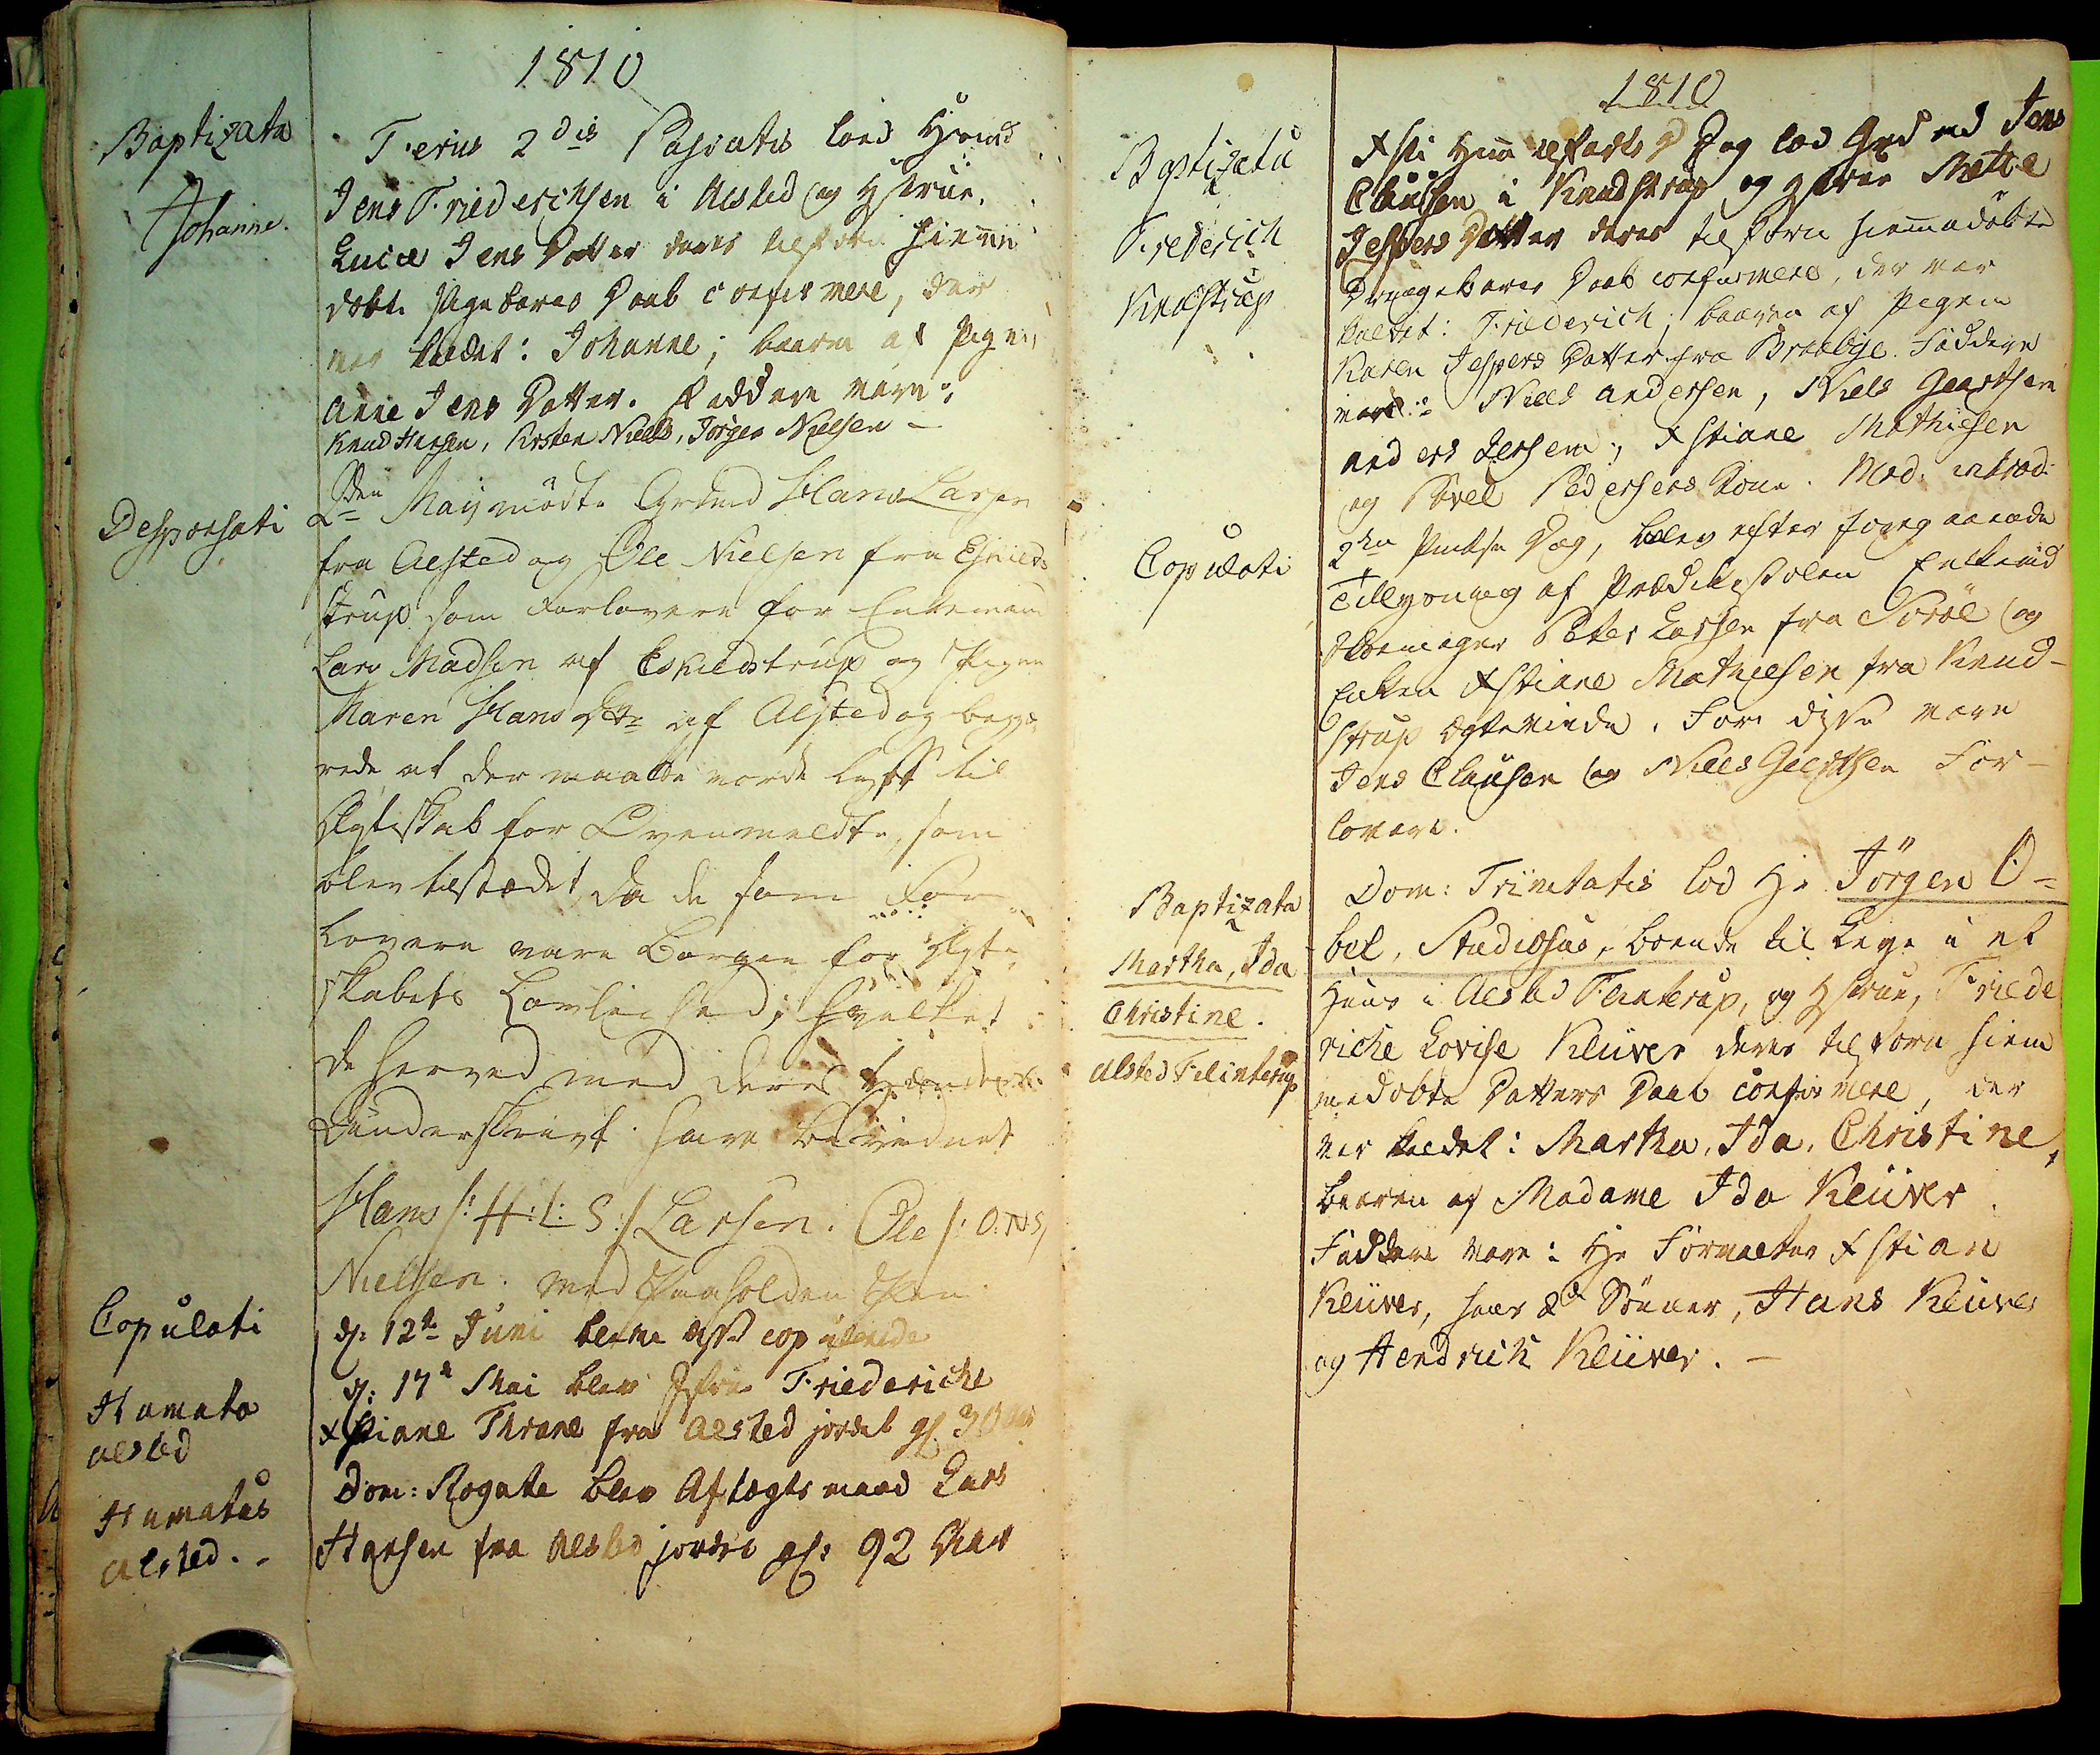Baptizata
Johanne
Desponsati
Copulati
Humata
Alsted
Hamatus
Alsted. -
1810
Feriis 2dis Pascatis loed Hsmd
Jens Friderichsen i Alsted og Hstrue.
Luice Jens Datter deres tilforn hiemme
døbet Pigebarns Daab confirmere, der
var kaldet: Johanne; baaren af Pigen
Anne Jens Datter. Faddere vare:
Knud Hansen, Krsten Niels, Jørgen Nielsen
2den Maij mødte Grdmd Hans Larsen
fra Alsted og Ole Nielsen fra Eskild¬
strup, som Forlovere for Enkemand
Lars Madsen af Eskildstrup og Pigen
Karen Hans Dtr af Alsted og begge¬
rede at der maatte vorde lyst til
Egteskab for Ovenmeldte, som
blev tilstædet da de som For¬
lovere vare Borgen for Ægte¬
skabets Lovlighed, hvilket
de herved med deres Hænders
Underskrivt have Bevidnet
Hans /: H: L: S :/ Larsen. Ole/: O: N. S/
Nielsen. med paaholden Pen
d: 12te Junii bleve disse copulerede
d: 17de Mai blev Jfrue Frideriches
Xstiane Thrane fra Alsted jordet gl 30 Aar
Dom: Rogate blev Aftægtsmand Lars
Hansen fra Alsted jordet gl: 92 Aar
Baptizatu
Frederich
Knudstrup
Copulati
Baptizata
Martha Ida
Christine
Alsted Flinterup
1810
Xsti Himmelfarts Dag lod Grdmd Jens
Claussen i Knudstyrup og Hstrue Mette
Jespers Datter deres tilforn hiemmedøbte
Drengebarns Daab confirmere, der var
kaldet: Friderich; baaren af Pigen
Karen Jespers Datter fra Braabye. Faddere
vare: Niels Anderssen, Niels Geertsen
Anders Jensen, Xstiane Mathisen
og Povel Pedersens Kone. Mod: introd:
2den Pindse Dag, blev efter foregaaende
Tillysning af Prædikestolen Enkemd
Skoemager Peter Larsen fra Sorøe og
Enken Xstiane Mathiesen fra Knud¬
strup Ægteviede. For disse vare
Jens Clausen og Niels Geertsen For¬
lovere.
Dom: Trinitatis lod Hr Jørgen O¬
bel Studiosus, boende til Leye i et
Huus i Alsted Flinterup, og Hstrue, Friede
riche Lovise Kluver deres tilforn hiem¬
medøbte Datters Daab confirmere, der
var kaldet: Martha, Ida, Christine,
baaren af Madame Ida Kluver
Faddere vare: Hr Forvalter Xstian
Kluver, hans 2de Sønner, Hans Kluver
og Hendrich Kluver.
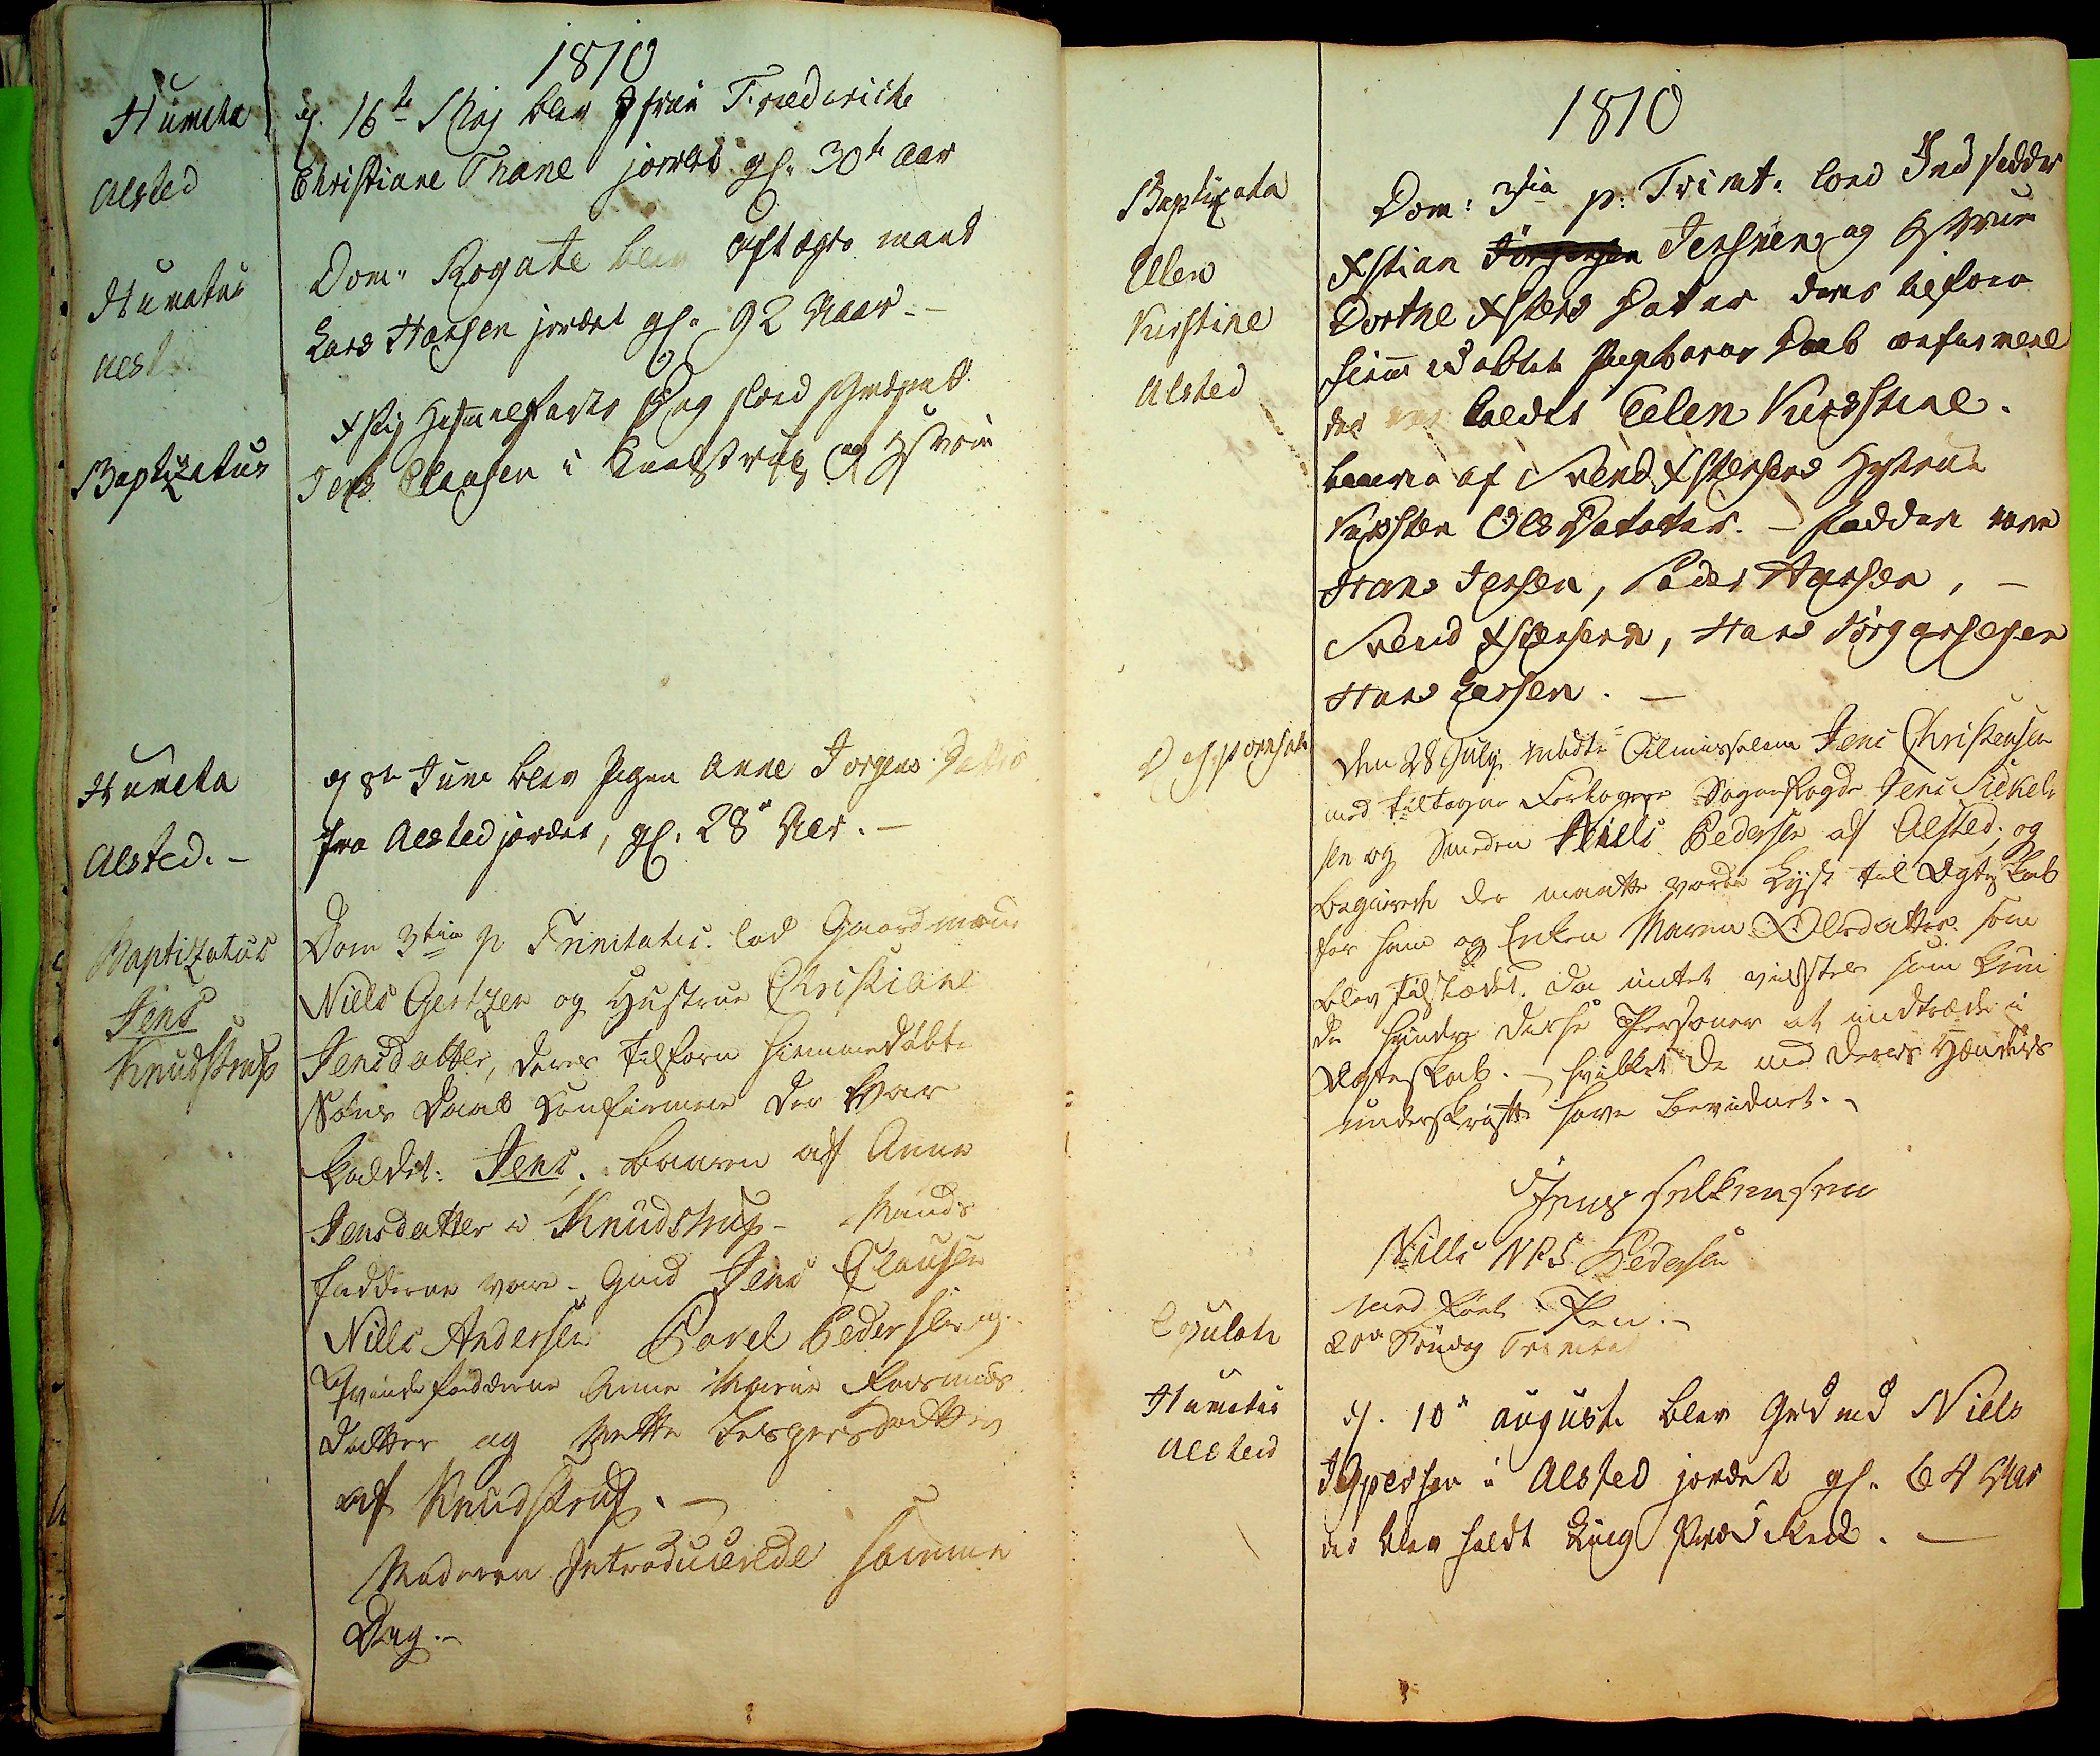Humata
Alsted
Humata
Alsted
Baptizatus
Humata
Alsted.
Baptizatus.
Jens
Knudstrup
1810
d. 16ta Maj blev Jfrue Frideriche
Christiane Thane jordet gl. 30t Aar.
Dom: Rogate blev Aftægtsmand
Lars Hansen jordet gl. 92 aar.
Xsti Himmelfarts Dag loed Grdmd
Jens Clausen i Knudstrup og Hstrue
d 8te Juni blev Pigen Anne Jørgens Datter
fra Alsted jordet gl. 28## Aar.
Dom 3tia p Trinitatis lod Gaardmand
Niels Gertzen og Hustrue Christiane
Jensdatter, deres tilforn hiemmedøbte
Søns Daab Confirmere der var
kaldet: Jens. baaren af Anne
Jensdatter i Knudstrup - Mands
Fadderne vare - Gmd Jens Clausen
Niels Andersen Povel Pedersen og
Qvinde Fadderne Anne Maren Rasmus
datter og Mette Jespersdatter
af Knudstrup.
Moderen Introducerede samme
Dag.
Baptizata
Ellen
Kirstine
Alsted
Desponsati
Copulati
Humatus
Alsted
1810
Dom: 3tia p: Trinit: loed Indsidder
Xstian Jørgensen Jensen og Hstrue
Dorthe Xstens Datter deres tilforn
shiemmedøbte Pigebarns Daab confirmere
det var kaldet Ellen Kirstine.
baaren af Svend Xstensens Hstrue
Kirsten Ols Datter. Faddere vare
Hans Jensen, Peder Hansen
Svend Xstensen, Hans Jørgensen
Hans Larsen.
Den 28 Julij mødte Almisselem Jens Christensen
med tiltagne Forlovere Sognefogde Jens Silken
sen og Smeden Niels Pedersen af Alsted og
begierede der maatte vorde Lyst til Ægteskab
for ham og Enken Maren Olsdatter, som
blev tilstædet, da intet vidste som kun
de hindre disse Personer at indtræde i
Ægteskab. hvilket de med deres Hænders
Underskrift have bevidnet.
Jens Silkensen
Niels NPS Pedersen
med ført Pen
20de Søndag Trinit
d. 10 Augusti blev Grdmd Niels
Jespersen i Alsted jordet gl. 64 Aar
der blev holdt Liig Prædiken